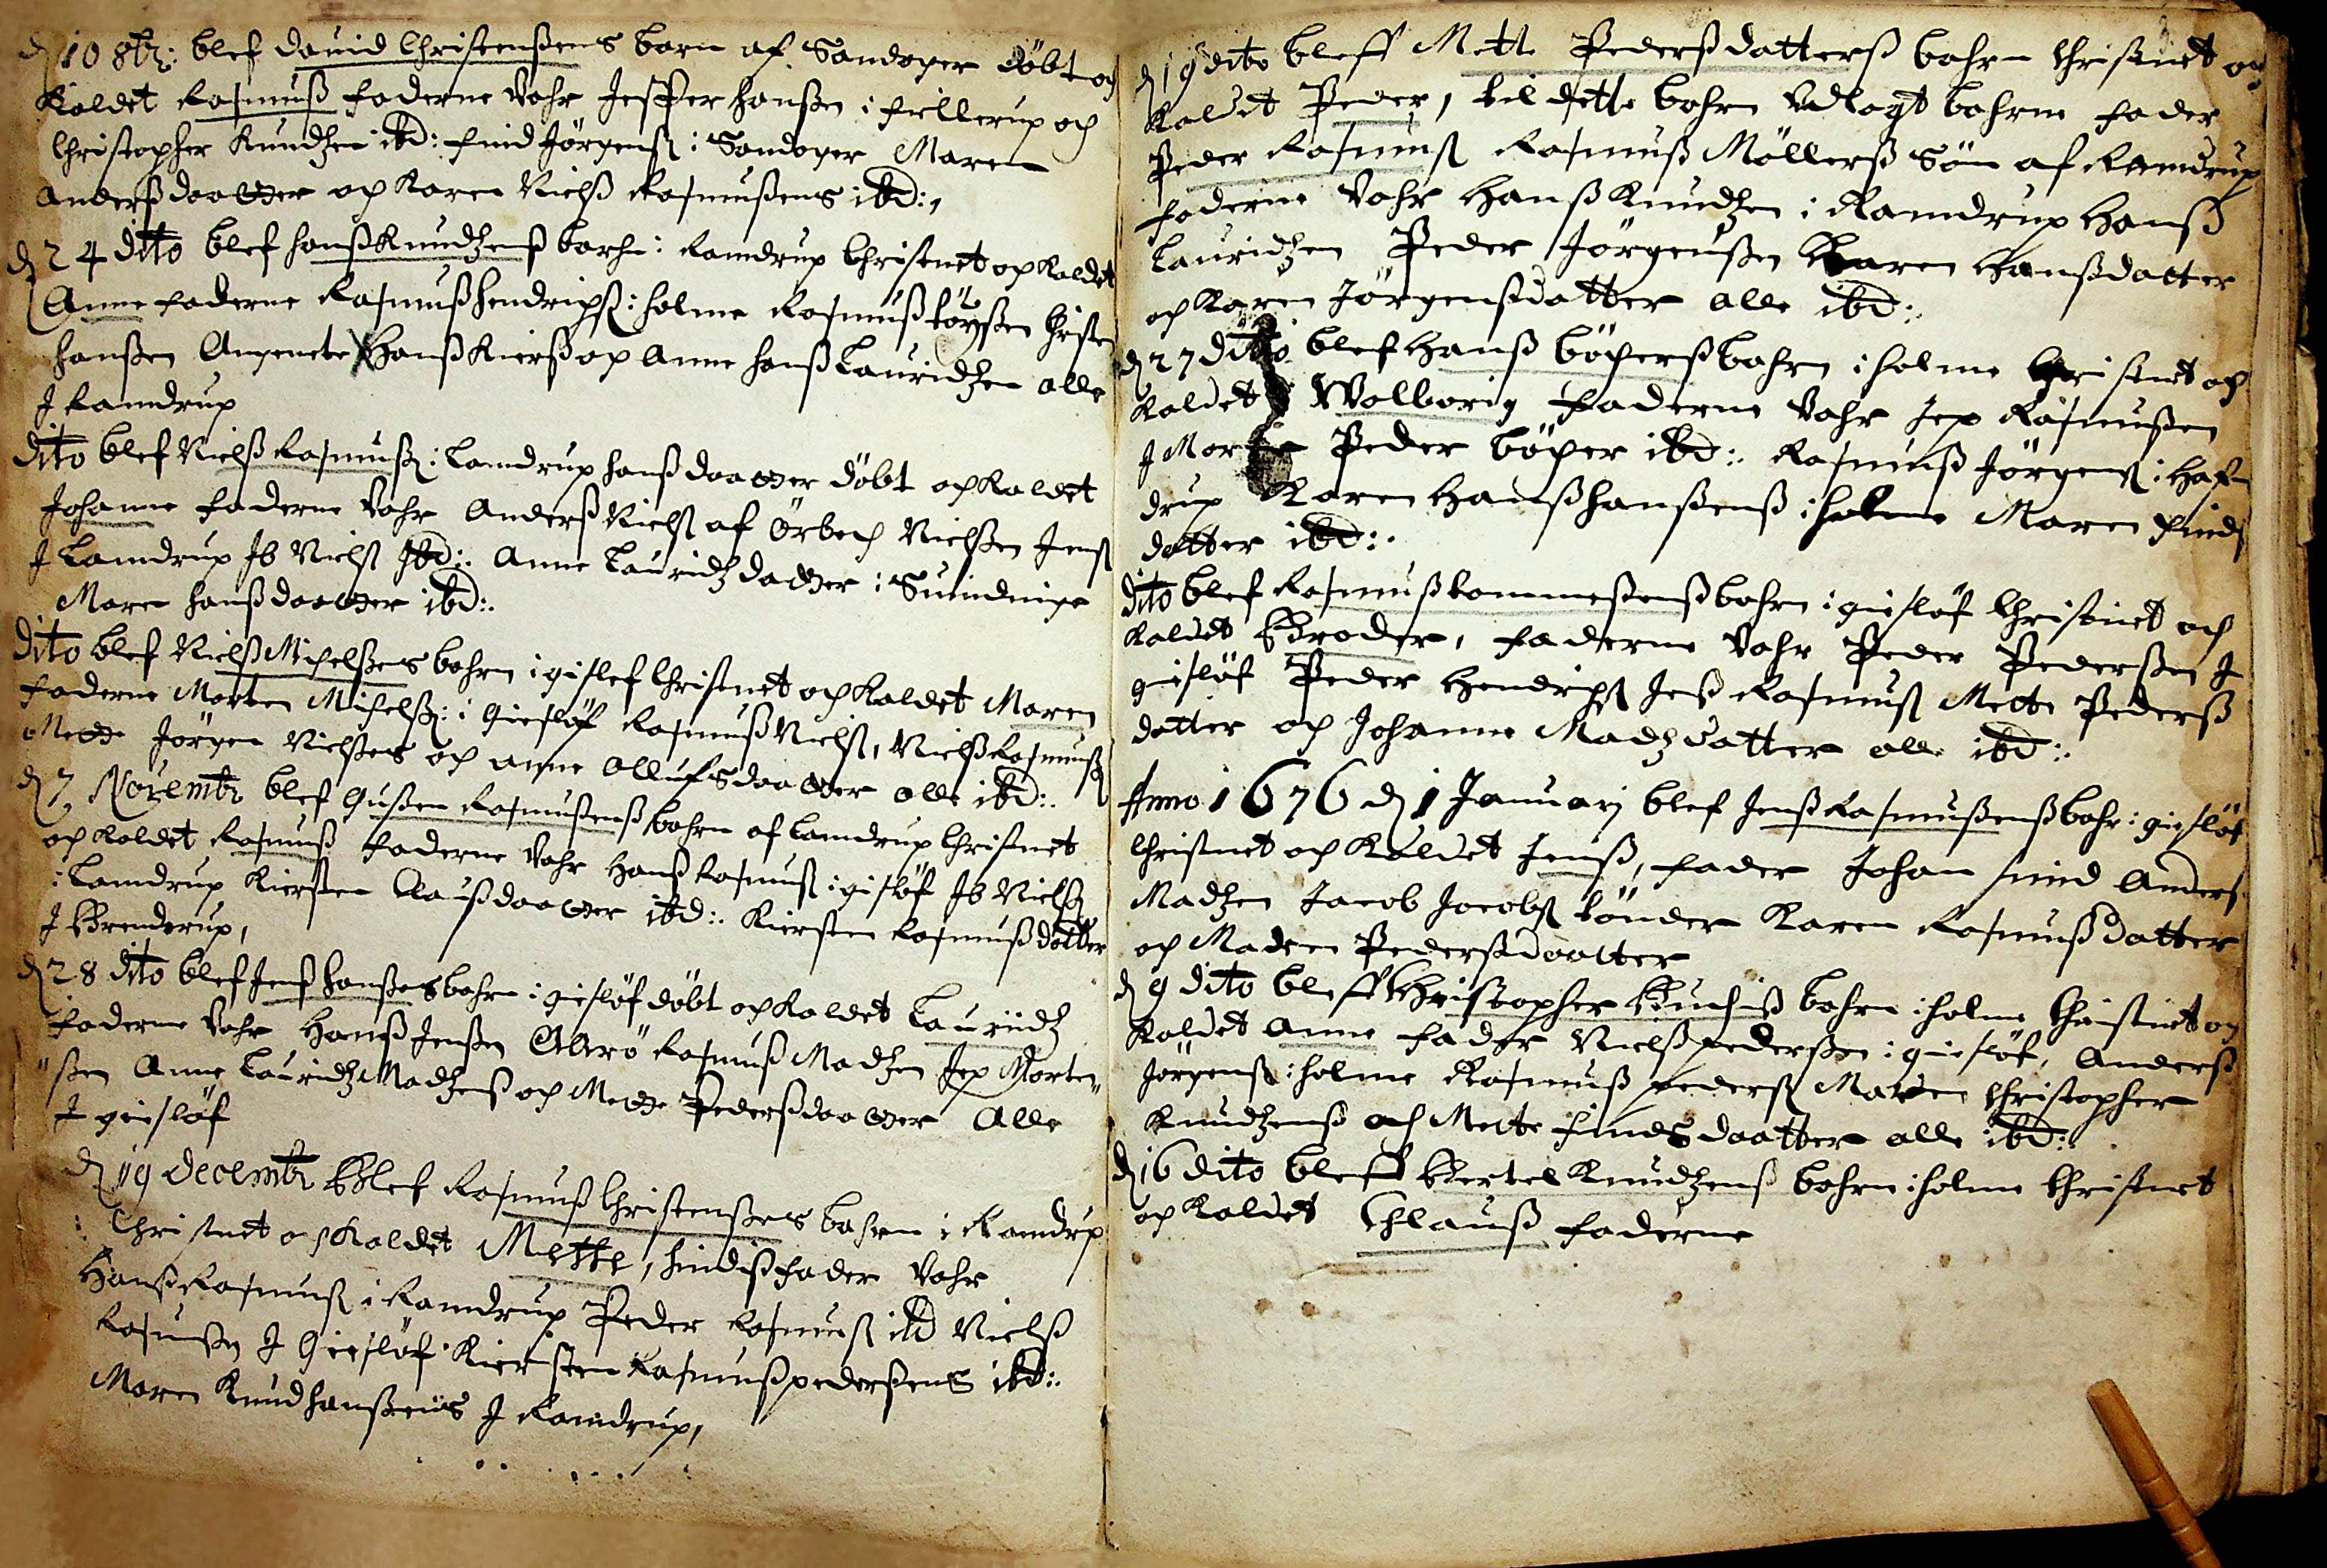d 10 8tr: blef Dauid Christenssens barn af Sandager døbt och
kaldet Rasmuss Faderne vahr Jesper Hanssen i Fiellerup och
Christopher Knudtzen ibd: Find Jørgenss i Sandager Maren
Andersdaatter och Karen Nielss Rasmussens ibd: 1
d 24 dito blef Hans Knudtzenss barh i Ramdrup Christenet och kaldet
Anne Faderne Rasmuss Hendrichs i Holme Rasmuss Tøy##ssen Christen
Hanssen Angenete Hanss Kierss och Anne Hanss Lauridtzen alle
I Ramdrup
Dito blef Nielss Rasmuss i Lamdrup Hanss daatter døbt och kaldet
Johanne Faderne vahr Anderss Nielss af Ørbech Nielssen Jenss
I Lamdrup Jb Nielss jbd:. Anne Lauridtz datter i Suindinge
Maren Hanssdaatter ibd:.
Dito blef Niels Michelsens bahrn i gislef Christnet och kaldet Maren
Faderne Morten Michelss i Giesløf Rasmuss Nielss, Nielss Rasmuss
Mette Jørgen Nielsens och Anne Ollufsdaatter alle ibd:.
d 7 Novembr blef Gussen Rasmussenss bahrn af Lamdrup Christnet
och kaldet Rasmuss Faderne vahr Hanss Rasmuss i gisløf Ib Nielss
I Lamdrup Kiersten Claussdaatter ibd:. Kiersten Rasmussdatter
I Brenderup,
d 28 dito blef Jenss Hanssens bahrn i Giesløf døbt och kaldet Lauriidtz
Faderne vahr Hanss Jenssen Aarø## Rasmuss Madtzen Jep Morten¬
ssen Anne Lauridtz Madtzens och Mette Pederssdatter Alle
I giesløf
. d 19 Decembr blef Rasmuss Christenssens bahrn i Ramdrup
Christnet och kaldet Mette, hindiss fader vahr
Hanss Rasmuss i Ramdrup Peder Rasmuss ibd Nielss
Rasmussen I Giesløf Kiersten Rasmuss pederssens ibd:.
Maren Knud Hanssens I Ramdrup,
3.
d 19 dito bleff Mette Pederssdatters bahrn Christnet och
kaldet Peder, til dette bahrn udlagt bahrne fader
Peder Rasmuss Rasmuss Møllerss Søn af Ramdrup
faderne vahr Hans Knudtzen i Ramdrup Hanss
Lauridtzen Peder Jørgenssen, Karen Hanssdatter
och Karen Jørgenssdatter alle ibd:.
d 27 dito blef Hanss Bøcherss bahrn i Holme Christnet och
kaldet Wolborig faderne vahr Jep Rasmussen
I Mor## Peder bøcher ibd:. Rasmuss Jørgenss i Haf¬
drup Karen Hanss Hanssenss i Holme Maren Finds
datter ibd:.
dito blef Rasmuss ##tommessenss bahrn i gisløf Christnet och
kaldet Broder, Faderne vahr Peder Pederssen I
giesløf Peder Hendrichs Jess Rasmuss Mette Pederss
datter och Johanne Madtzdatter alle ibd:.
Anno 1676 d 1 Januarij blef Jenss Rasmussenss bahr i giesløf
christnet och kaldet Jenss, Fader Johan smid Anders
Madtzen Jacob Jocobs tø##der Karen Rasmussdatter
och Maren## Pedersdaatter
d 9 dito bleff Christopher ##Buchiss bahrn i holme Christnet och
kaldet Anne Fader Niels pedersen i Giesløf, Anderss
Jørgenss i holme Rasmuss pederss Maren Christopher
Knudtzens och Mette Findsdaatter alle ibd:.
d 16 dito bleff Bertel Knudtzenss bahrn i holme Christnet
och kaldet Chlauss faderne
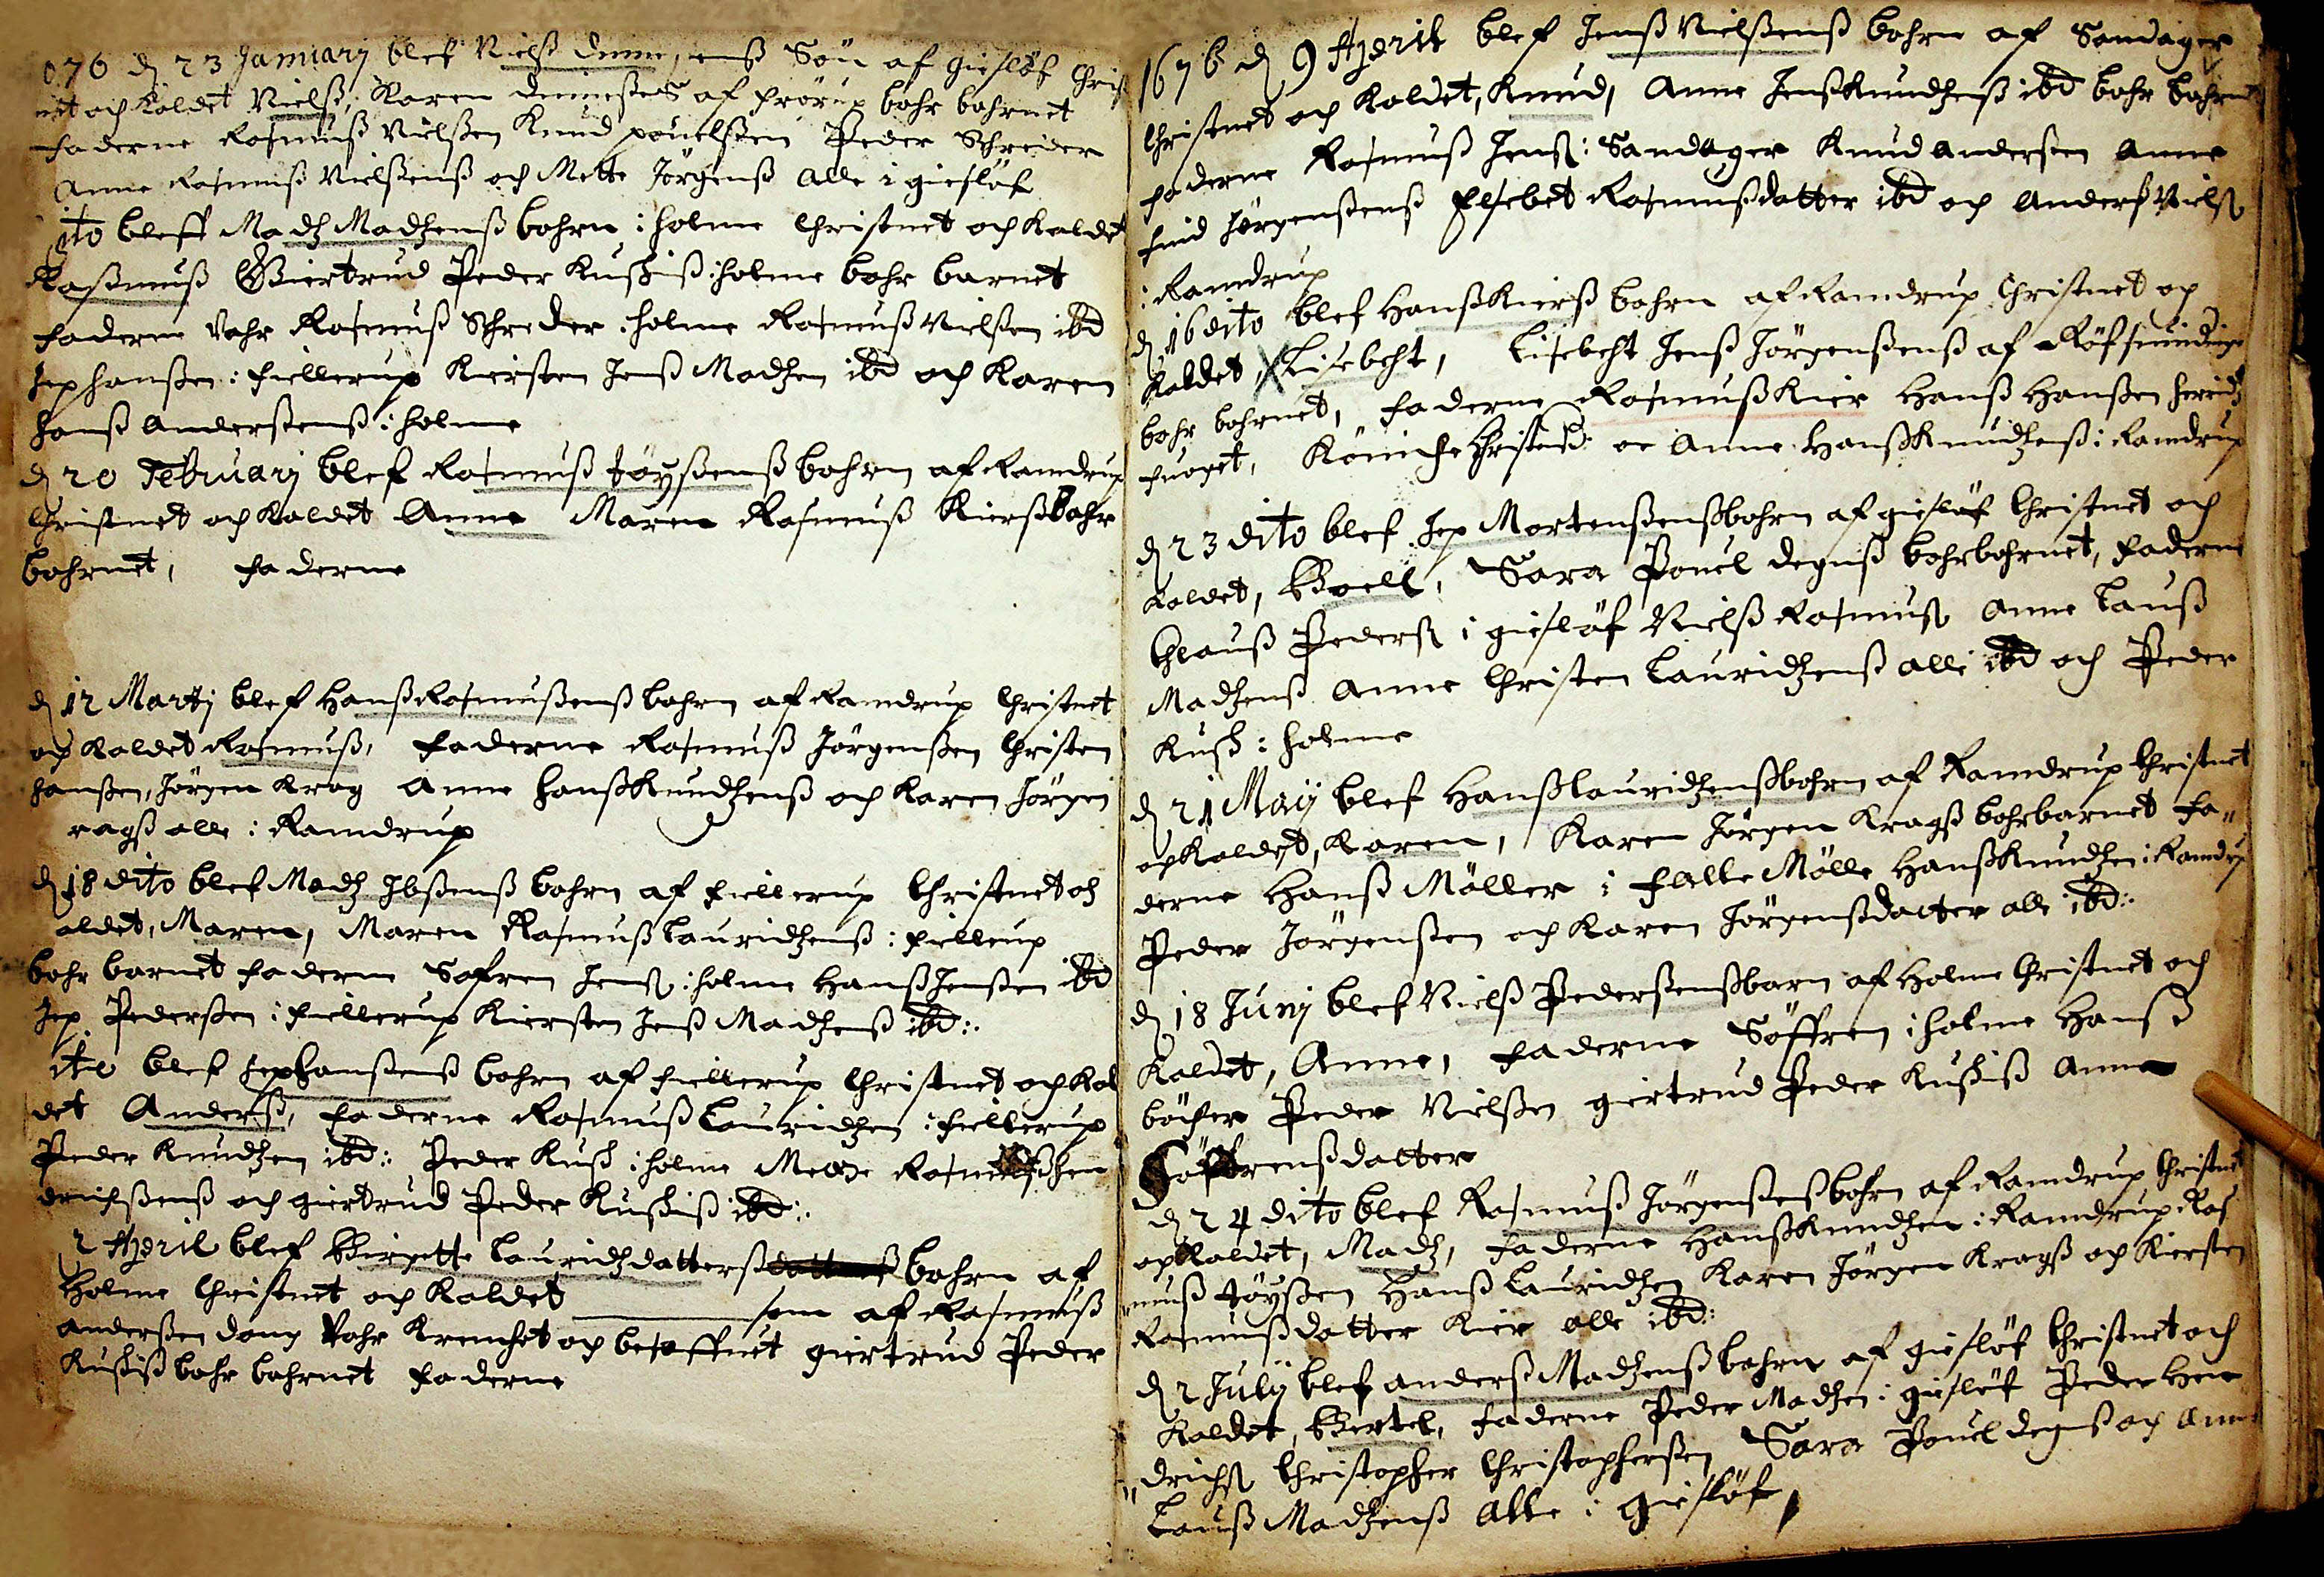## 76 d 23 Januarij blef Nielss Dee##enss Søn af Giesløf Christ
net och kaldet Nielss, Karen Dei##esses af Frørup bahr bahrnet
Faderne Rasmuss Nielssen Knud Pouelssen, Peder Schreder
Anne Rasmuss Nielssenss och Mette Jørgenss alle i giesløf
ito bleff Madtz Madtzenss bahrn i holme christnet och kaldet
Rasmuss Giertrud Peder Kuschiss i holme bahr barnet
Faderne vahr Rasmuss Schreder i holme Rasmuss Nielssen ibd
Jep hanssen i Frellerup Kiersten Jenss Madtzen ibd och Karen
hans anderssenss i Holme
d 20 Februarij blef Rasmuss Jørgssenss## bahrn af Ramdrup
christnet och kaldet Anne Maren Rasmuss Kierss bahr
bahrnet, Faderne
d 12 Martij blef Hanss Rasmussenss bahrn af Ramdrup christenet
och kaldet Rasmuss, Faderne Rasmuss Jørgenssen Christen
hansen, Jørgen Krog Anne Hanss Knudtzenss och Karen Jørgen
rogs alle i Ramdrup
d 18 dito blef Madtz Ibssenss bahrn af Frellerup Christenet och
kaldet Maren, Maren Rasmuss Lauridtzens i Fiellerup
bahr barnet Faderne Søfren Jenss i holme Hanss Jensen ibd
Jep Pederssen i Fiellerup Kirsten Jenss Madtzens ibd:.
ito blef Jep Hansenss bahrn af Fiellerup christnet och kal
det Anderss, Faderne Rasmuss Lauridtzen i Fiellerup
Peder Knudtzen ibd:. Peder Kusch## i holme Mette Rasmuss Hen
drichssenss och Giertrud Peder Kuskis ibd:.
2 April blef Birgette Lauridtzdatterss ## bahrn af
Holme Christnet oc kaldet _ som af Rasmuss
Andersen dong vahr Krenchet ox besoffuet Giertrend Peder
bahr bahrnet Faderne
v.
1676 d 9 Apriit blef Jens Nielssenss bahrn af Sandager
Christnet og Kaldet Knud, Anne Jens Knudtzens ibd. bahr bahrnet
Faderne Rasmuss Jenss i Sandager Knud Anderssen Anne
smid Jørgenssenss Elsebet Rasmussdatter ibd och Anders Nelss
i Ramdrup
d. 16 dito blef Hanss Kierss bahrn af Ramdrup Christnet og
Kaldet Lisebeht, Lisebecht Jenss Jørgenssenss af Røfseuindinge
nahr bahrnet, Faderne Rasmuss Kier Hanss Hanssen herrids
fuoget, Køniche## Christensd: oc Anne Hanss Knudtzenss i Ramdrup
d 23 dito blef Jep Mortenssens bahrn af giesløf Christnet och
kaldet, Boell, Sara Pouel Degnss bahr bahrnet, Faderne
Chlauss Pederss i giesløf Nielss Rasmuss Anne Lauss
Madtzens Anne Christen Lauridtzenss alle ibd och Peder
Kusk## i Holme
d 21 Maij blef Hanss Lauridzenss bahrn af Ramdrup Christnet
och Kaldet, Karen, Karen Jørgen Kragss bahr bahrnet Fa¬
derne Hanss Møller i Falle Mølle Hanss Knudtzen i Ramdrup
Peder Jørgenssen och Karen Jørgenssdatter alle ibd:.
d 18 Junij blef Nielss Pederssenss barn af Holme Christnet och
Kaldet, Anne, Faderne Søffren i holme Hanss
bøcher Peder Nielssen, giertrud Peder Kuskiss## Anne
Søffrenssdatter
d 24 dito blef Rasmuss Jørgenssenss bahrn af Ramdrup Christnent
och Kaldet, Madtz, Faderne Hanss Knudtzen i Ramtrup Ras
muss tøyssen## Hanss Lauidtzen, Karen Jørgen Krogs och Kiersten
Rasmussdatter Kier alle ibd.:
d 2 Julij blef anderss Madtzenss bahrn af giesløf Christnet och
Kaldet, Bertel, Faderne Peder Madtzen i giesløf Peder Hen
drichs Christopher Christopherssen Sara Pouel degnss og Anne
Lauss Madtzenss Alle i Giesløf